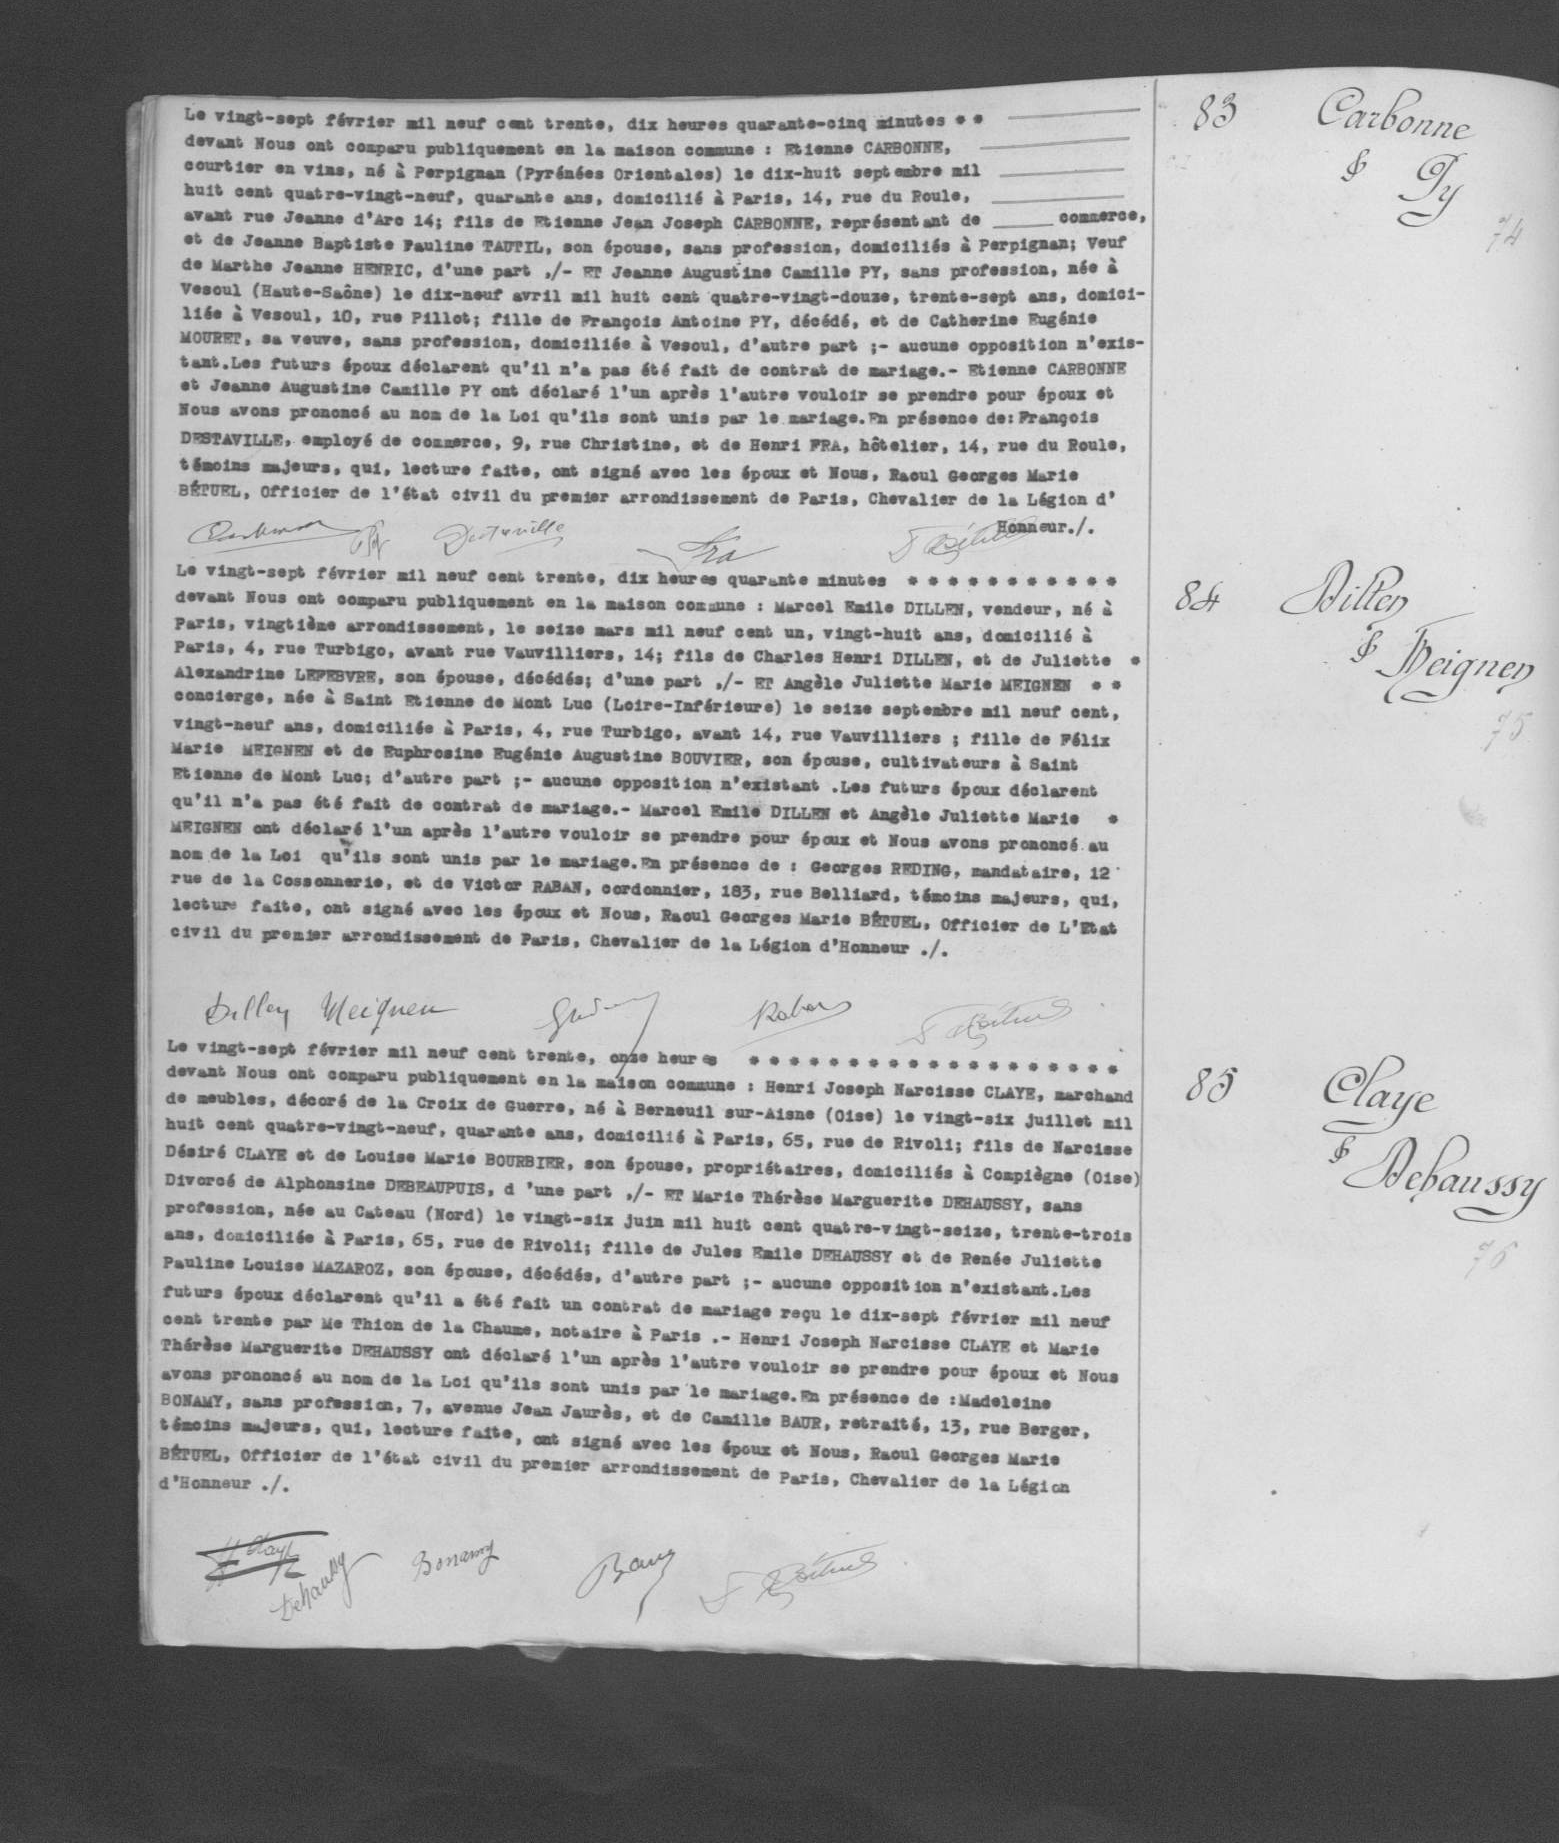Le vingt sept février mil neuf cent trente, dix heure quarante-cinq minutes * * -
devant Nous ont comparu publiquement en la maison commune: Etienne CARBONNE, -
courtier en vins, né à Perpignan (Pyrénées orientales) le dix-huit septembre mil -
huit cent quatre-vingt-neuf, quarante ans, domicilié à Paris, 14, rue du Roule, -
avant rue Jeanne d'Arc 14; fils de Etienne Jean Joseph CARBONNE, représentant de -commerce,
et de Jeanne Bptiste Pauline TAUTIL, son épous, sans profession domiciliés à Perpignan; Veuf
de Marthe Jeanne HENRIC, d'une part ,/-ET Jeanne Augustine Camille PY, sans profession, née à
Vesoul (Haute-Saône) le dix-neuf avril mil huit cent quatre-vingt-douze, trente-sept ans, domici-
liée à Vesoul, 10, rue Pillot; fille de François Antoine PY, décédé, et de Catherine Eugénie
MOURRET, sa veuve, sans profession, domiciliée à Vesoul, d'autre part ;-aucune opposition n'exis-
tant. Les futurs époux déclarent qu'il n'a pas été fait de contrat de mariage .-Etienne CARBONNE
et Jeanne Augustine Camille PY ont déclaré l'un après l'autre vouloir se prendre pour époux et
Nous avons prononcé au nom de la Loi qu'ils sont unis par le mariage. En présence de: François
DESTAVILLe, employé de commerce, 9, rue Christine, et de Henri FRA, hôtelier, 14, rue du Roule,
témoins majeurs, qui, lecture faite, ont signé avec les époux et Nous, Raoul Georges Marie
BETUEL, Officier de l'état civil du premier arrondissement de Paris, Chevalier de la Légion d'
Honneur ./.
Le vingt-sept février mil neuf cent trente, dix heures quarante minutes ****
devant Nous ont comparu publiquement en la maison commune: Marcel Emile DILLEM, vendeur, né à
Paris, vingtième arrondissement, le seize mars mil neuf cent un, vingt-huit ans, domicilié à
Paris, 4, rue Turbigo, avant rue Vauvilliers, 14; fils de Charles Henri DILLEM, et de Juliette *
Alexandrine LEFEBRE, son épouse, décédés; d'une part ,/-ET Angèle Juliette Marie MEIGNEN * *
concierge, née à Saint Etienne de Mont Luc (Loire-Inférieure) le seize septembre mil neuf cent,
vingt-neuf ans, domiciliée à Paris, 4, rue Turbigo, avant 14, rue Vauvilliers; fille de Félix
Marie MEIGNEN et de Euphrosine Eugénie Augustine BOUVIER, son épouse, cultivateurs à Saint
Etienne de Mont Luc; d'autre part ;-aucune oppositin n'existant. Les futurs époux déclarent
qu'il n'a pas été fait de contrat de mariage .-Marcel Emile DILLEN et Angèle Juliette Marie *
MEIGNEN ont déclaré l'un après l'autre vouloir se prendre pour époxu et Nous avons prononcé au
nom de la Loi qu'ils sont unis par le mariage. En présence de: Georges REDING, mandataire, 12
Rue de la Cossonnerie, et de Victor RABAN, cordonnier, 183, rue Belliard, témoins majeurs, qui,
lecture faite, ont signé avec les époux et Nous, Raoul Georges Marie BETUEL, Officier de L'Etat
civil du premier arrondisement de Paris, Chevalier de la Légion d'Honneur ./.
Le vingt-sept février mil neuf cent trente, onze heures ****
devant Nous ont comparu publiquement en la maison commune: Henri Joseph Narcisse CLAYE, marchand
de meubles, décoré de la Croix de Guerre, né à Berneuil sur-Aisne (Oise) le vingt-six juillet mil
huit cent quatre-vingt-neuf, quarante ans, domicilié à Paris, 65, rue de Rivoli; fils de Narcisse
Désiré CLAYE et de Louise Marie BOURBIER, son épouse, propriétaires, domiciliés à Compiègne (Oise)
Divorcé de Alphonsine DEBEAUPUIS, d'une part ,/-ET Marie Thérèse Marguerite DEHAUSSY, sans
profession, née au Cateau (Nord) le vingt-six juin mil huit cent quatre-vingt-seize, trente-trois
ans, domiciliée à Paris, 65, rue de Rivoli; fille de Jules Emile DEHAUSSY et de Renée Juliette
Pauline Louise MAZAROZ, son épouse, décédés, d'autre part ;-aucune opposition n'existant. Les
futurs époux déclarent qu'il a été fait un contrat de mariage reçu le dix-sept février mil neuf
cent trente par Me Thion de la Chaume, notaire à Paris .-Henri Joseph Narcisse CLAYE et Marie
Thérèse Marguerite DEHAUSSY ont déclaré l'un après l'autre vouloir se prendre pour époux et Nous
avons prononcé au nom de la Loi qu'ils sont unis par le mariage. En présence de :Madeleine
BONAMY, sans profession, 7, avenue Jean Jaurès, et de Camille BAUR, retraité, 13, rue Berger,
témoins majeurs, qui, lecture faite, ont signé avec les époux et Nous, Raoul Georges Marie
BETUEL, Officier de l'état civil du premier arrondissement de Paris, Chevalier de la Léion
d'Honneur.
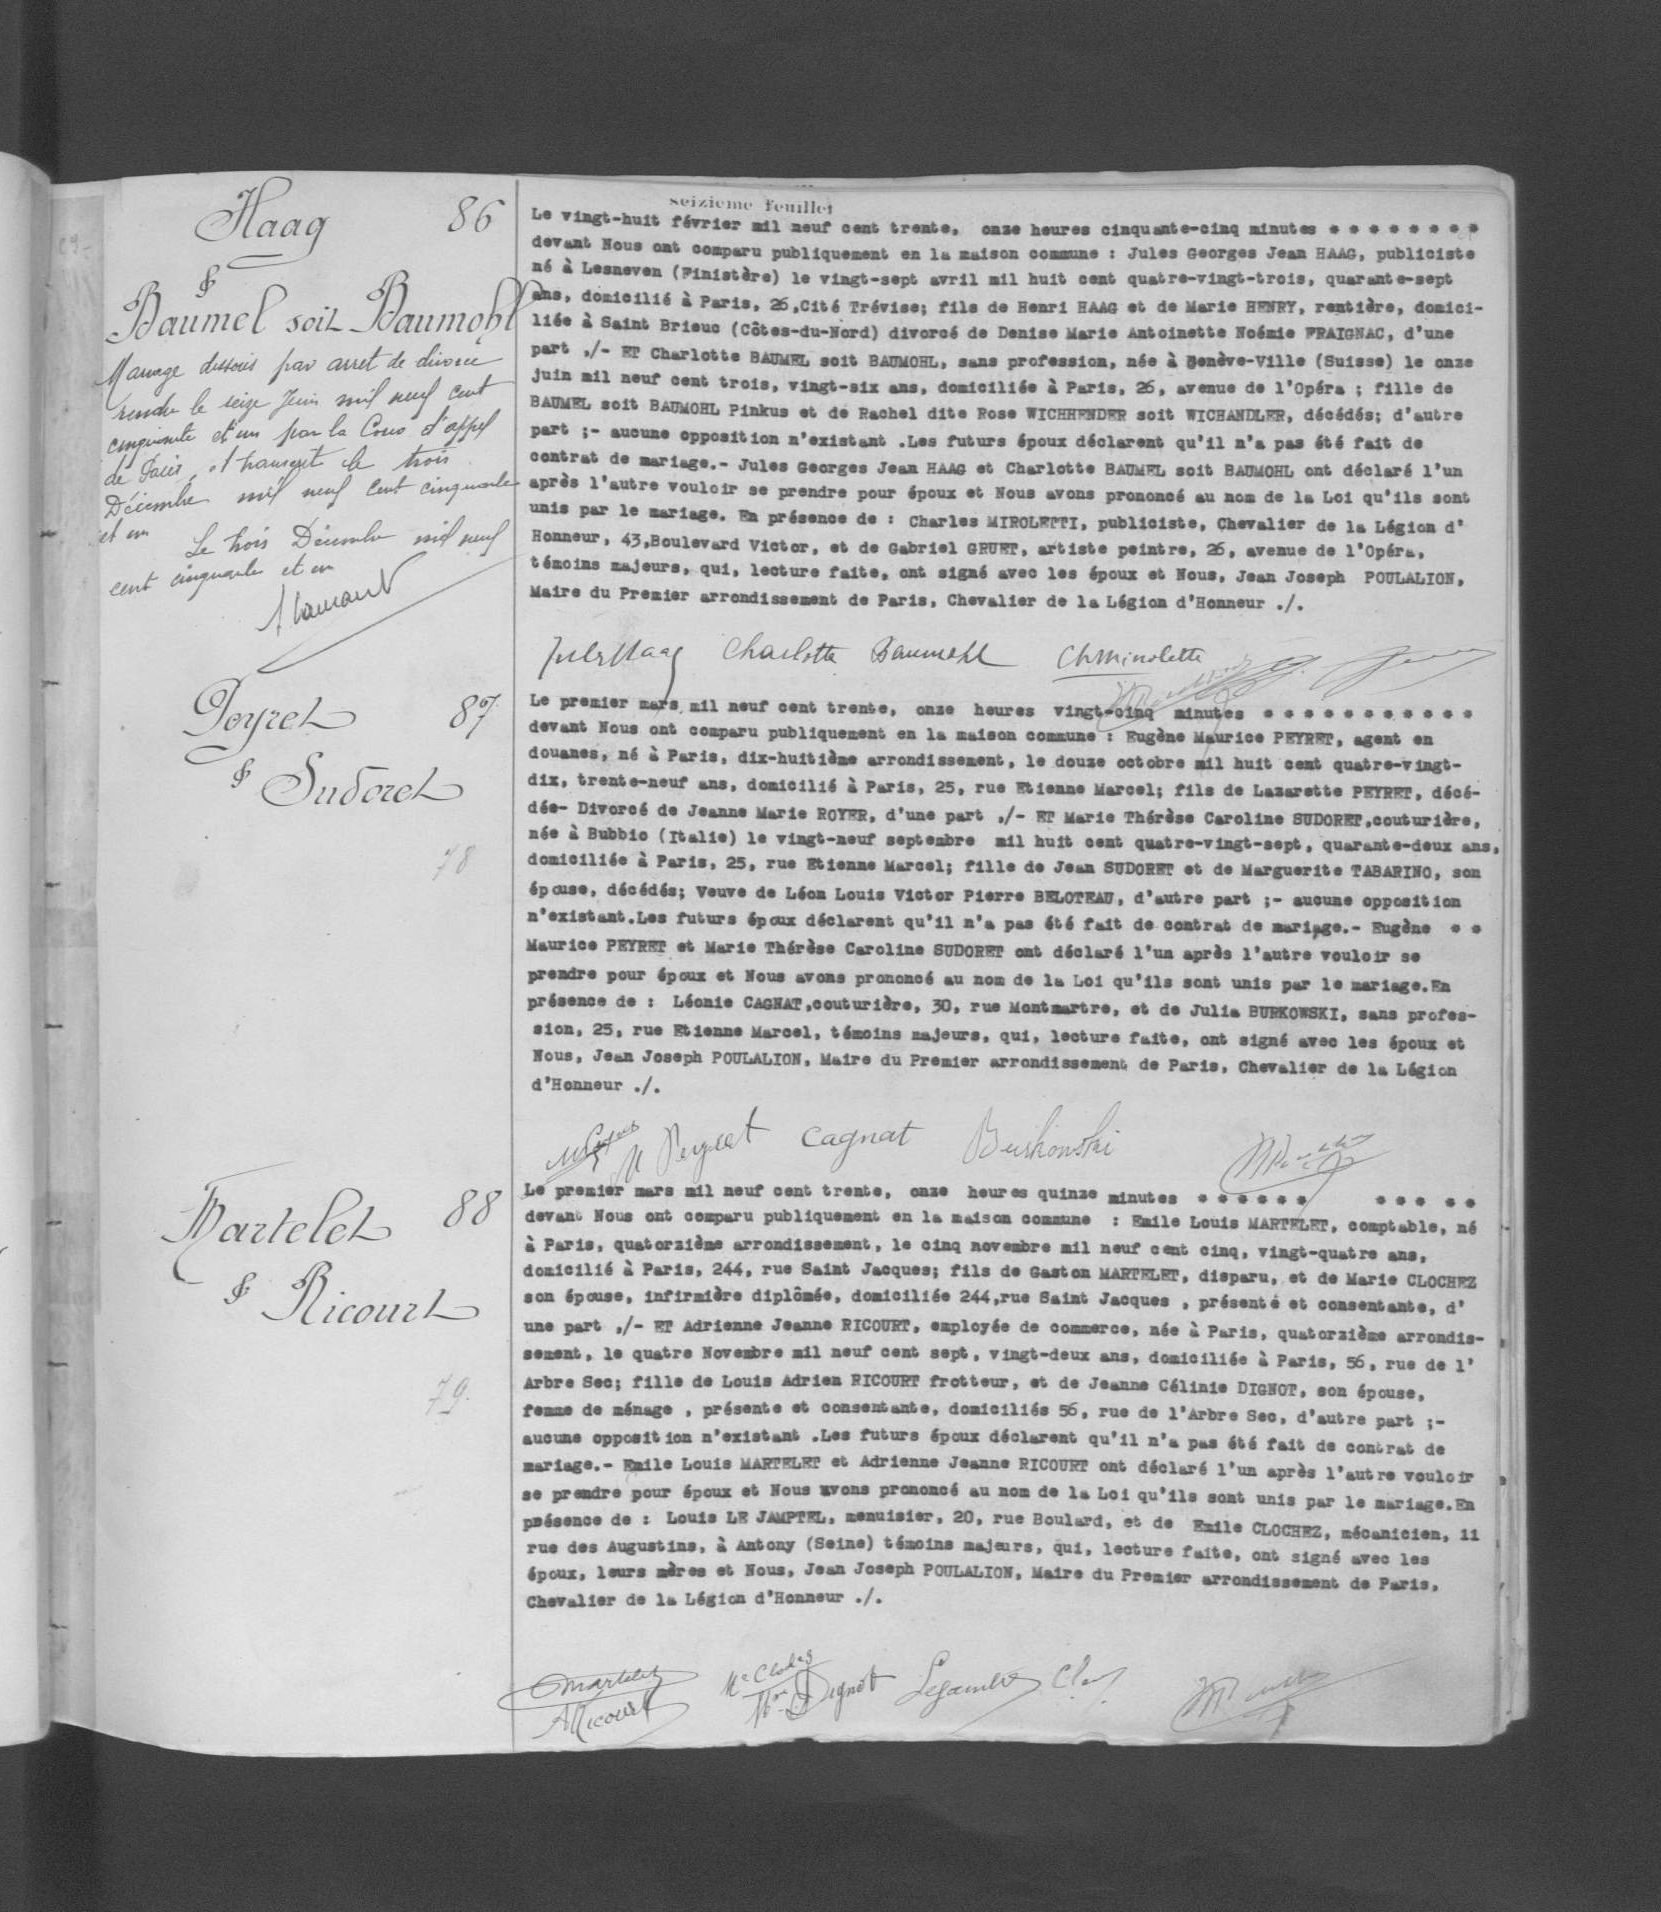Le vingt-huit février mil neuf cent trente, onze heures cinquante-cinq minutes ****
devant Nous ont comparu publiquement en la maison commune: Jules Georges Jean HAAG, publiciste
né à Lesneven (Finistère) le vingt-sept avril mil huit cent quatre-vingt-trois, quarante-sept
ans, domicilié à Paris, 26, Cité Trévise; fils de Henri HAAG et de Marie HENRY, rentière, domici-
liée à Saint Brieuc (Côtes-du-Nord) divorcé de Denise Marie Antoinette Noémie FRAIGNAC, d'une
part ,/-ET Charlotte BAUMEL soit BAUMOHL, sans profession, née à Genève-Ville (Suisse) le onze
juin mil neuf cent trois, vingt-six ans, domiciliée à Paris, 26, avenue de l'Opéra; fille de
BAUMEL soit BAUMOHL Pinkus et de Rachel dite Rose WICHENDER soit WICHANDLER, décédés; d'autre
part ;-aucune opposition n'existant. Les futurs époux déclarent qu'il n'a pas été fait de
contrat de mariage .-Jules Georges Jean HAAG et Charlotte BAUMEL soit BAUMOHL ont déclaré l'un
après l'autre vouloir se prendre pour époux et Nous avons prononcé au nom de la Loi qu'ils sont
unis par le mariage. En présence de: Charles MIROLETTI, publiciste, Chevalier de la Légion d'
Honneur, 43, Boulevard Victor, et de Gabriel GRUET, artiste peintre, 26, avenue de l'Opéra,
témoins majeurs, qui, lecture faite, ont signé avec les époux et Nous, Jean Joseph POULALION,
Maire du Premier arrondissement de Paris, Chevalier de la Légion d'Honneur ./.;
Le premier mars mil neuf cent trente, onze heures vingt-cinq minutes ****
devant Nous ont comparu publiquement en la maison commune: Eugène Maurice PEYRET, agent en
douanes, né à Paris, dix-huitième arrondissement, le douze octobre mil huit cent quatre-vingt-
dix, trente-neuf ans, domicilié à Paris, 25, rue Etienne Marcel; fils de Lazarette PEYRET, décé-
dée-Divorcé de Jeanne Marie ROYER, d'une part ,/-ET Marie Thérèse Caroline SUDORET, couturière,
née à Bubbio (Italie) le vingt-neuf septembre mil huit cent quatre-vingt-sept, quarante-deux ans,
domiciliée à Paris, 25, rue Etienne Marcel; fille de Jean SUDORET et de Marguerite TABARINO, son
épouse, décédés; Veuve de Léon Louis Victor Pierre BELOTEAU, d'autre part ;-aucune opposition
n'existant. Les futurs époux déclarent qu'il n'a pas été fait de contrat de mariage .-Eugène * *
Maurice PEYRET et Marie Thérèse Caroline SUDORET ont déclaré l'un après l'autre vouloir se
prendre pour époux et Nous avons prononcé au nom de la Loi qu'ils sont unis par le mariage. En
présence de: Léonie CAGNAT, couturière, 30, rue Montmartre, et de Julia BUEKOWSKI, sans profes-
sion, 25, rue Etienne Marcel, témoins majeurs, qui, lecture faite, ont signé avec les époux et
Nous, Jean Joseph POULALION, Maire du Premeir arrondissement de Paris, Chevalier de la Légion
d'Honneur ./.
Le premier mars mil neuf cent trente, onze heures quinze minutes ****
devant Nous ont comparu publiquement en la maison commune: Emile Louis MARTELET, comptable, né
à Paris, quatorzième arrondissement, le cinq novembre mil neuf cent cinq, vingt-quatre ans,
domicilié à Paris, 244, rue Saint Jacques; fils de Gaston MARTELET, disparu, et de Marie CLOCHEZ
son épouse, infirmière diplômée, domiciliée 244, rue Saint Jacques, présente et consentante, d'
une part ,/-ET Adrienne Jeanne RICOURT, employée de commerce, née à Paris, quatorzième arrondis-
sement, le quatre Novembre mil neuf cent sept, vingt-deux ans, domiciliée à Paris, 56 rue de l'
Arbre Sec; fille de Louis Adrien RICOURT frotteur, et de Jeanne Célinie DIGNOT, son épouse,
femme de ménage, présente et consentante, domicilié 56, rue de l'Arbre Sec, d'autre part ;-
aucune opposition n'existant. Les futurs époux déclarent qu'il n'a pas été fait de contrat de
mariage .-Emile Louis MARTELET et Adrienne Jeanne RICOURT ont déclaré l'un après l'autre vouloir
se prendre pour époux et Nous avons prononcé au nom de la Loi qu'ils sont unis par le mariage. En
présence de: Louis LE JAMPTEL, menuisier, 20, rue Boulard, et de Emile CLOCHEZ, mécanicien, 11
rue des Augustins, à Antony (Seine) témoins majeurs, qui, lecture faite, ont signé avec les
époux, leurs mères et Nous, Jean Joseph POULALION, Maire du Premier arrondissement de Paris,
Chevalier de la Légion d'Honneur ./.